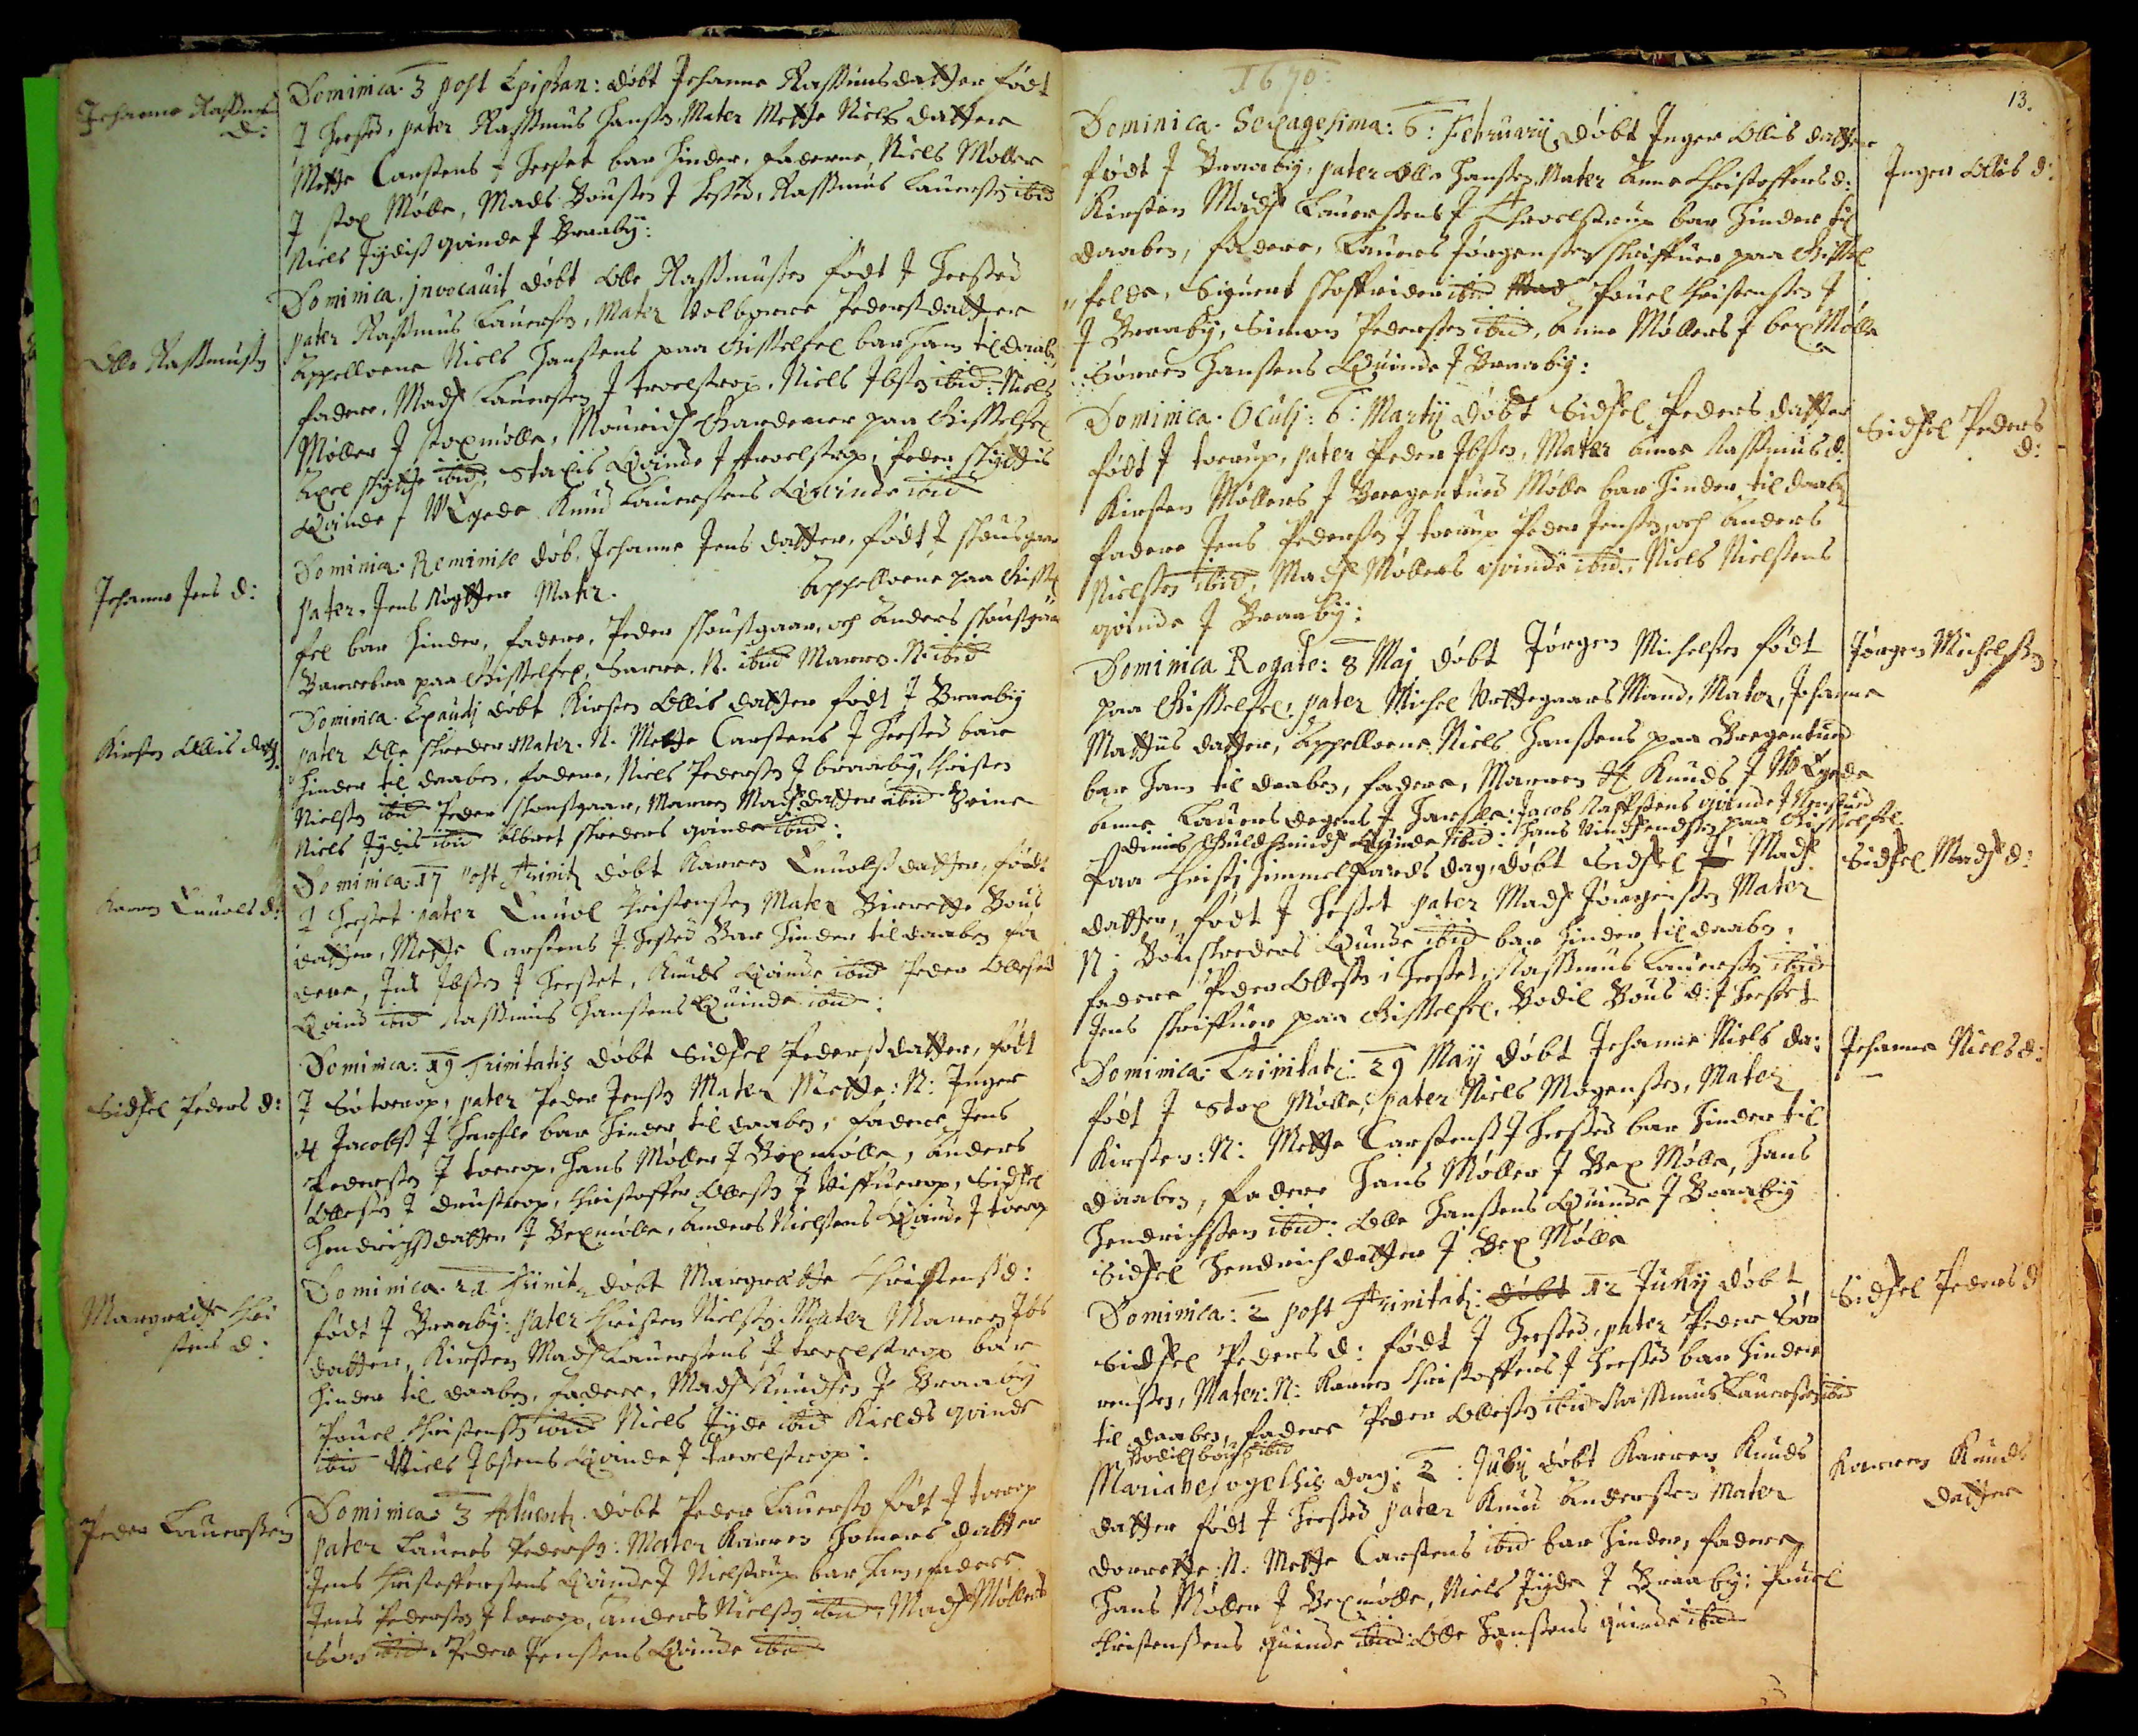Johanne Rasmus¬
d:
Dominica. 3 post Epiphan: døbt Johanne Rasmusdatter, født
J Heesed, Pater Rassmus Hansen, Mater Mette Nielsdatter 
Mette Carstens i Heeset bar hinder, Faderne, Niels Møller
J Stox Mølle, Mads Bousen J Hesed, Rassmus Laursen ibid.
Niels Jydis Qvinde J Braaby:
Olle Rasmusen
Dominica. Invocauit døbt Olle Rasmusen født J Heesed
Pater Rasmus Laursen, Mater Valborre Pedersdatter
Appelloene Niels Hansens paa Gisselfel bar ham til Daaben.
Faderne Mads Laursen J Troelstrup, Niels Jbsen ibid. Niels
Møller J Stox Mølle, Mourids Gardener paa Gisselfel
Axel Skytte ibid. Staxis## Qvinde J Troelstrup, Peder skyttis
Qvinde J V.Egede, Knud Laursens Qvinde ibid.
Johanne Jens d: 
Dominica Reminise døbt Johanne Jens datter født J Skousgaar,
Pater Jens Røgtter Mater.
Appelloene paa Gissel
fel bar hinder, Faderne, Peder Skousgaar och Anders Skousgaar
Barrabra paa Gisselfel, Sarra N. ibid. Marren N. ibid.
Kirsten Ollis dat##
Dominica Exaudi døbt Kirsten Ollis datter født J Braaby 
Pater Olle Skræder Mater N., Mette Carstens i Heesed bar 
hinder til daaben. Faderne, Niels Pedersen J Braaby, Christen
Nielsen ibid. Peder Skousgaar, Maren Madzdatter ibid. Seine##
Niels Jydis ibid. Albert Skieders qvinde ibid:
Karren Enuols d: 
Dominica: 17 post trinit døbt Karren Enuols datter, født
J Heeset, Pater Enuol Christensen Mater Birrette Bous
datter, Mette Carstens J Heesed Bar hinder til daaben; Fa¬
dere, Jens Jbsen J Herset, Knuds Qvinde ibid. Peder Larsens
Qvinde ibid. Rassmus Hansens Qvinde ibid.
Sidsel Peders d:
Dominica 19 Trinitatis døbt Sidsel Pedersdatter, født
J Søtoerup, Pater Peder Jensen, Mater Mette N:, Jnger 
H## Jacobs J Hasle## bar hinder til daaben; Fadere Jens
Pedersen J Toerup, Hans Møller J Bex Mølle, Anders
Ollesen J Drustrop, Christopher Ollesen i Vistuerop, Sidsel
Hendrichsdatter J Bexmølle, Anders Nielsens Qvinde J Toerup.
Margrethe Chri
stens d:
Dominnica. 22 Trinit døbt Margræthe Christens d:
født J Braaby. Pater Christen Nielsen. Mater Maren Jbs 
datter, Kirsten Madz Laursens J Troelstrop bar
hinder til daaben. Fadere, Mads Knudzen J Braaby,
Pouel Christensen ibid. Niels Jyde ibid. Kields qvinde
ibid Niels Jbsens Qvinde J Troelstrop:
Peder Laursen 
Dominica 3 Aduent døbt Peder Laursen, født J Toerup.
Pater Laurs Pedersen. Mater Karren Homaes datter
Jens Christoffersens Qvinde J Nielstrup bar ham, Fadere
Jens Pedersen J Toerop, Anders Nielsen ibid. Mads Møllers
søn ibid. Peder Jensens Qvinde ibid.
1670:
Dominica. Sexagesima: 6: February døbt Jnger Ollis datter
født J Braaby, Pater Olle Hansen, Mater Anna Christoffersd: 
Kirsten Mads Lauersens J Throelstrup bar hinder til
daaben, Fadere, Lauers Jørgensen skriffuer paa Gissel
felde, Siguert Skoffrider ibid. Pouel Christensen
J Braaby, Simon Pedersen ibid. Anne Møllers i Bex Mølle
Sørren Hansens Quinde J Braaby:
13.
Inger Ollisd:
Dominica. Oculi: 6: Marty døbt Sidsel Peders datter
født J Toerup, Pater Peder Ibsen, Mater Anna Rassmusd: 
Kirsten Møllers J Beregentued Mølle bar hinder til daaben
Fadere Jens Pedersen J Toerup Peder Jensøn, och Anders 
Nielsen ibid. Mads Møllers quinde ibid. Niels Nielsens
quinde J Braaby:
Sidsel Peders
d: 
Dominica Rogate: 8 Maj døbt Jørgen Michelsen født
paa Gisselfel, Pater Michel Urttegaards Mand, Mater, Johanne 
Mattiis datter, Appelloene Niels Hansens paa Bregentued 
bar ham til daaben; Fadere, Marren H Knuds J V. Egede
Anne Lauers Degens J Harsle: Jacob Naflsens## quinde J Neestued,
Dines Guldsmieds Quinde ibid: Hans Vindpendsen## paa Gisselfel.
Jørgen Michelsen
Paa Christi Himmelfards dag: døbt Sidsel J## Mads
datter, født J Heset, Pater Mads Jørgensen, Mater
N: Boe Skreders Quinde ibid. bar hinder til daaben.
Fadere Peder Ollesen i Heeset, Rassmus Lauersen ibid.
Jens Skriffuer paa Gisselfel, Bodil Bous d: J Heeset.
Sidsel Madsd:
Dominica: Trinitati. 29 May døbt Johanne Nielsda:
født J Stox Mølle, Pater Niels Mogensen, Mater 
Kirsten: N: Mette Carstens J Heesed bar hinder til 
daaben, Fadere Hans Møller J Bex Mølle, Hans
Hendrichsen ibid. Olle Hansens Quinde J Braaby.
Sidsel Hendrichdatter J Bex Mølle.
Johanne Nielsd:
Dominica: 2 post trinitat: døbt 12. Juny døbt
Sidsel Peders d: født J Heesed, Pater Peder Sør
rensen, Mater: N: Karren Christoffers J Heesed bar hinder
til daaben, Fadere Peder Ollesen ibid. Rassmus Lauersøns ibid.
Bodil Bous ibid.
Sidsel Pedersd.
Maria besøgelsis dag: 2: July døbt Karren Knuds
datter født J Heesed Pater Knud Andersøn Mater 
Dorrette: N: Mette Carstens ibid. bar hinder, Fadere
Hans Møller J Bexmølle, Niels Jyde J Braaby, Pouel
Christensens quinde ibid. Olle Hansens quinde ibid.
Karren Knuds 
datter
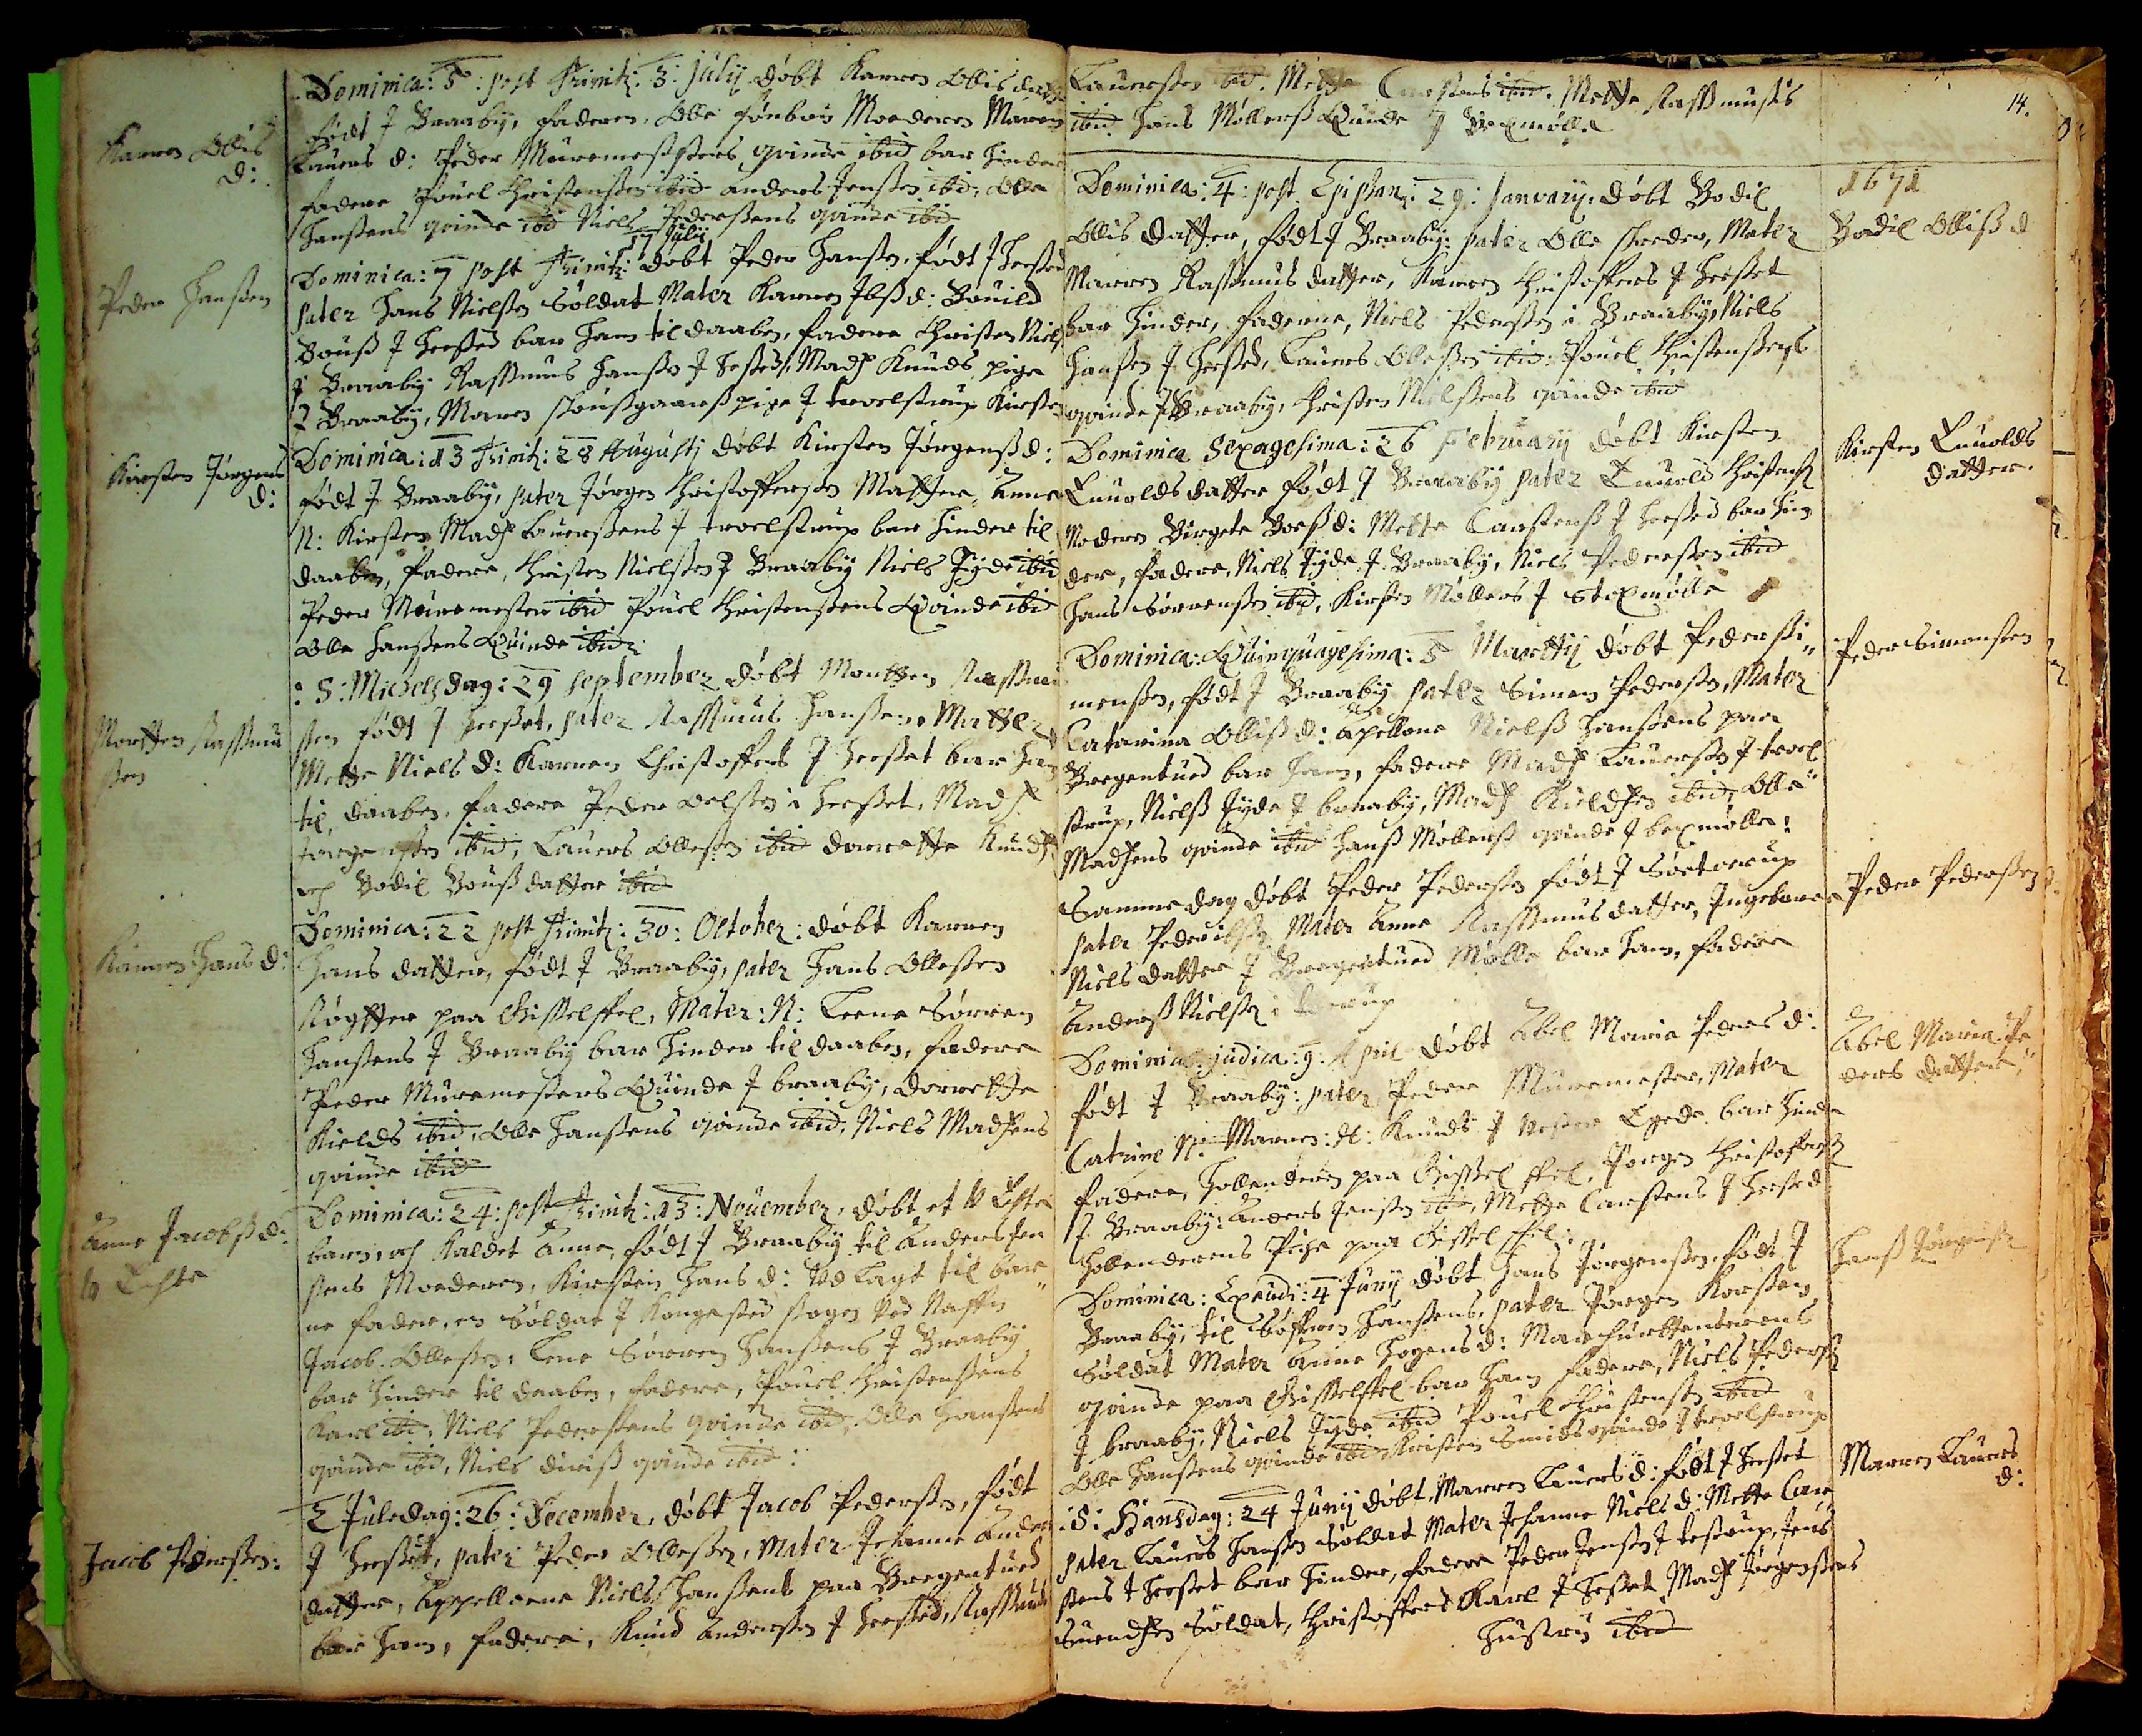Karren Ollis
d:
Dominica: 5. Post Trinit 3. July døbt Karren Ollis datter
døbt J Braaby. Faderen, Olle Fønboe##. Moderen Maren##
Laurs d: Peder Murermeststers qvinde ibid. bar hinder.
Fadere Pouel Christensen ibid. Anders Jensen ibid. Olle
Hansens quinde ibid, Niels Pedersens quinde ibid.
Peder Hansen
17 July
Domiinica: 7 Post Trinit: døbt Peder Hansen født J Heesed.
Pater Hans Nielsen Søldat. Mater Karren Jbsd: Bouild
Bousd. J Heesed bar ham til daaben, Fadere: Christen Niels
J Braaby, Rassmus Hansen J Hesed, Mads Knuds pige
J Braaby, Maren Skousgaars pige J Troelstrup Kirsten.
Kirsten Jørgens 
d:
Dominica: 13 Trinit: 28 Augustj døbt Kirsten Jørgensd:
født J Braaby. Pater Jørgen Christoffersen. Matter Anne
N: Kirsten Mads Lauersen J Troelstrup bar hinder til
Daaben, Fadere, Christen Nielsen J Braaby, Niels Jyde ibid.
Peder Murermester ibid. Pouel Christensens Qvinde ibid.
Olle Hansens Quinde ibid.
Mortten Rassmus
sen 
:S: Michelsdag: 29 September døbt Morten Rassmus
sen født J Heeset. Pater Rassmus Hansen. Matter
Mette Niels d: Karren Christoffers J Heeset bar ham
til daaben. Fadere: Peder Oelsen i Heeset, Mads
Jørgensen ibid. Laurids Ollesen ibid. Dorrette Knuds
och Bodil Bousdatter ibid.
Karren Hans d: 
Dominica: 22: post Trinit: 30: October: døbt Karren 
Hans datter, født J Braaby, Pater Hans Ollesen 
Røgtter paa Gisselffel, Mater: N: Leene Sørren 
Hansens J Braaby bar hinder til daaben. Fadere 
Peder Muremesters Quinde J Braaby; Dorrette 
Kields ibid. Olle Hansens quinde ibid. Niels Madzens
quinde ibid.
Anne Jacobsd:
U Echte 
Dominica: 24: post Trinit: 13: Nouember, døbt et U Echte
barn och kaldet Anne, født J Braaby til Anders Jen
sen Moederen, Kirstin Hans d: Udlagt til bar¬
ne fader, en Søldat J Kongested Sogn ved Naffn
Jacob Ollesen, Lene Sørren Hansens J Braaby
bar hinder til daaben, Fadere, Pouel Christensens
karl ibid. Niels Pedersens qvinde ibd. Olle Hansens
qvinde ibd, Niels Diris qvinde ibid:
Jacob Pedersen:
2 Juledag: 26: December døbt Jacob Pedersen, født 
J Heeset, Pater Peder Ollesen, Mater Johanne Anders 
datter, Appelloena Niels Hansens paa Bregentued 
bar ham, Fadere, Knud Andersen J Heesed, Rassmus
Laursen ibid, Mette Carstens ibid. Mette Rassmusd.
ibid. Hans Møllers Quinde J Bexmølle.
14.
Dominiica: 4: post Epiphan: 29. Jauary døbt Bodil
Ollis Datter, født J Braaby: Pater Olle Skræder, Mater
Marren Rassmusdatter, Karren Christoffers J Heeset
bar hinder, Fadere, Niels Pedersen i Braaby, Niels
Hansen J Heeset, Lauers Ollesen ibid. Pouel Christensens
quinde J Braaby, Christen Nielsens quinde ibid.
1671
Bodil Ollis d
Dominica. Sexagesima: 26 February døbt Kirsten
Enuoldsdatter født J Braaby Pater Enuold Christensen
Moderen Birgite Boesd: Mette Carstens i Heeset bar hin¬
der, Fadere, Niels Jyde J Braaby, Niels Pedersen ibid.
Hans Sørrensen ibid. Kirsten Møllers J Stoxmølle.
Kirsten Enuolds 
datter. 
Dominica Quinquagesima: 5 Marty døbt Peder Si¬
monsen født J Braaby. Pater Simon Pedersen. Mater 
Catarina Ollisd: Apellone Niels Hansens paa
Bregentued bar ham, Fadere Mads Lauersen J Troel
strup. Niels Jyde J Braaby, Mads Kieldsen ibid. Olle
Madsens qvinde ibid. Hans Møllers qvinde J Bex mølle;
Peder Simonsen
Samme dag døbt Peder Pedersen født J Søetoerup 
Pater: Peder Ibsen, Mater: Anne Rassmusdatter, Ingeborre 
Nielsdatter J Bregentued Mølle bar ham. Fadere
Anders Nielsen i Toerup.
Peder Pedersen
Dominica Judica: 9: April døbt Abel Maria Pedersd: 
født J Braaby. Pater: Peder Muremester, Mater 
Catrine N: Marren H Knuds J Vester Egede bar hinder. 
Fadere, Hollænderen paa Gisselfel, Jørgen Christoffersen 
J Braaby, Anders Jensen ibid. Mette Carstens J Heesed
Hollænderens Pige paa Gisselfel:
Abel Maria Pe 
ders datter.
Dominica Exaudi: 4 Juny døbt Hans Jørgensen, født J
Braaby, til Sørren Hansens, Pater Jørgen Karsten##
Søldat Mater: Anne Jørgensd: Marhurbtenterens##
qvinde paa Gisselfel bar ham Fadere, Niels Pedersen 
J Braaby, Niels Jyde ibid. Pouel Christensen ibid.
Olle Hansens qvinde ibid. Kristen Smids qvinde J Troelstrup.
Hans Jørgensen
S: Hansdag: 24 Juny døbt Marren Lauersd. født J Heeset
Pater Lauers Hansen Søldat Mater Johanne Nielsd. Mette Car¬ 
stens i Heeset bar hinder, Fadere Peder Jensen J Testrup, Jens 
Frandsen## Søldat, Christopher Karl i Hesed, Mads Jørgensens
Hustrue ibid.
Marren Lauers 
d: